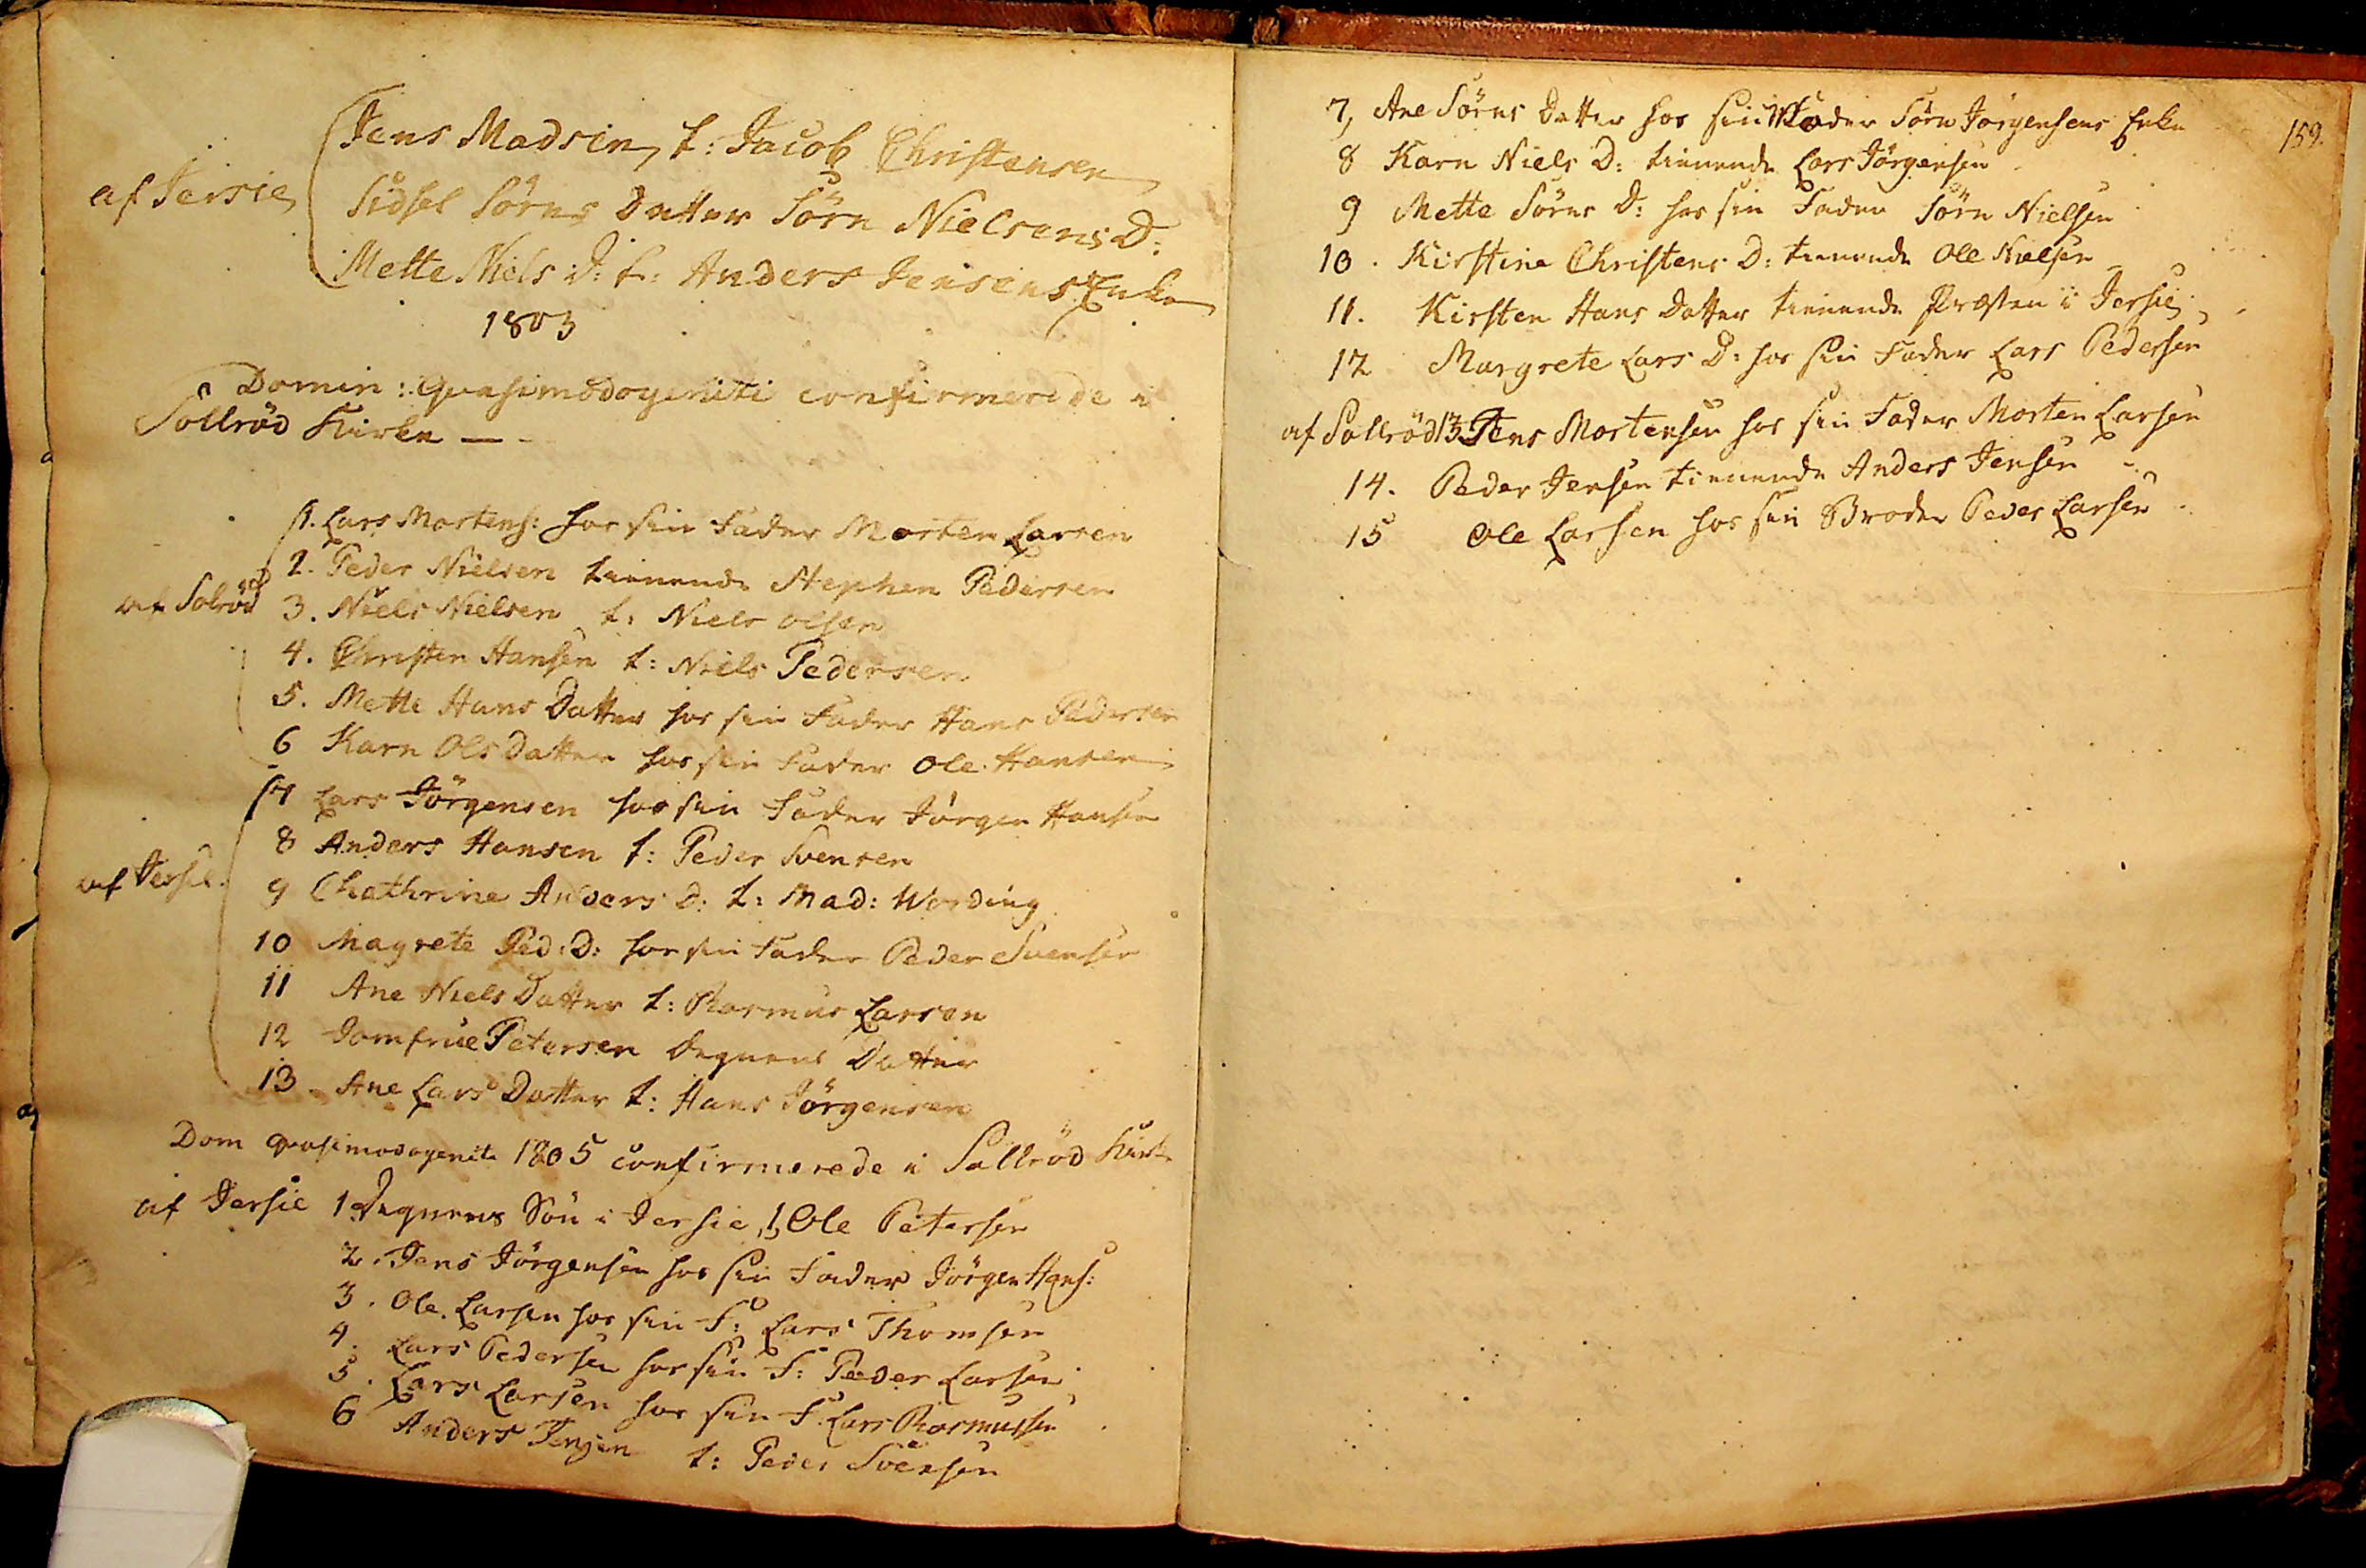Jens Madsen, t: Jacob Christensen
af Jersie Sidsel Sørns Datter Sørn Nielsens D:
Mette Niels D: t: Anders Jensens Enke
1803
Domin: Qvasimodogeniti Confirmerede i
Sollrød Kirke --
1. Lars Mortens: hos sin Fader Morten Larsen
2. Peder Nielsen tienende Stephen Pedersen
af Solrød 3. Niels Nielsen t: Niels Olsen
4. Christen Hansen t: Niels Pedersen
5. Mette Hans Datter hos sin Fader Hans Pedersen
6 Karn Ols Datter hos sin Fader Ole Hansen
7 Lars Jørgensen hos sin Fader Jørgen Hansen
8 Anders Hansen t: Peder Svensen
af Jersie 9. Chathrine Anders D: t: Mad: Wording
10. Magrete Ped: D: hos sin Fader Peder Svensen
11 Ane Niels Datter t: Rasmus Larsen
12 Jomfrue Petersen Degnens Datter
13. Ane Lars Datter t: Hans Jørgensen
Dom Qvasimodogeniti 1805 Confirmerede i Sollrød Kirke
af Jersie 1. Degnens Søn i Jersie, 1, Ole Petersen
2. Jens Jørgensen hos sin Fader Jørgen Hans:
3. Ole Larsen hos sin F: Lars Thomsen
4. Lars Pedersen hos sin F: Peder Larsen
5. Lars Larsen hos sin F: Lars Rasmussen
6. Anders Jensen t: Peder Svensen
159.
7, Ane Sørns Datter hos sin Moder Sørn Jørgensens Enke
8 Karn Niels D: tienende Lars Jørgensen
9 Mette Sørns D: hos sin Fader Sørn Nielsen
10. Kirsten Christens D: tienende Ole Nielsen
11. Kirsten Hans Datter tienende Præsten i Jersie
12. Margrete Lars D: hos sin Fader Lars Pedersen
af Solrød 13 Jens Mortensen hos sin Fader Morten Larsen
14. Peder Jensen tienende Anders Jensen
15 Ole Larsen hos sin Broder Peder Larsen
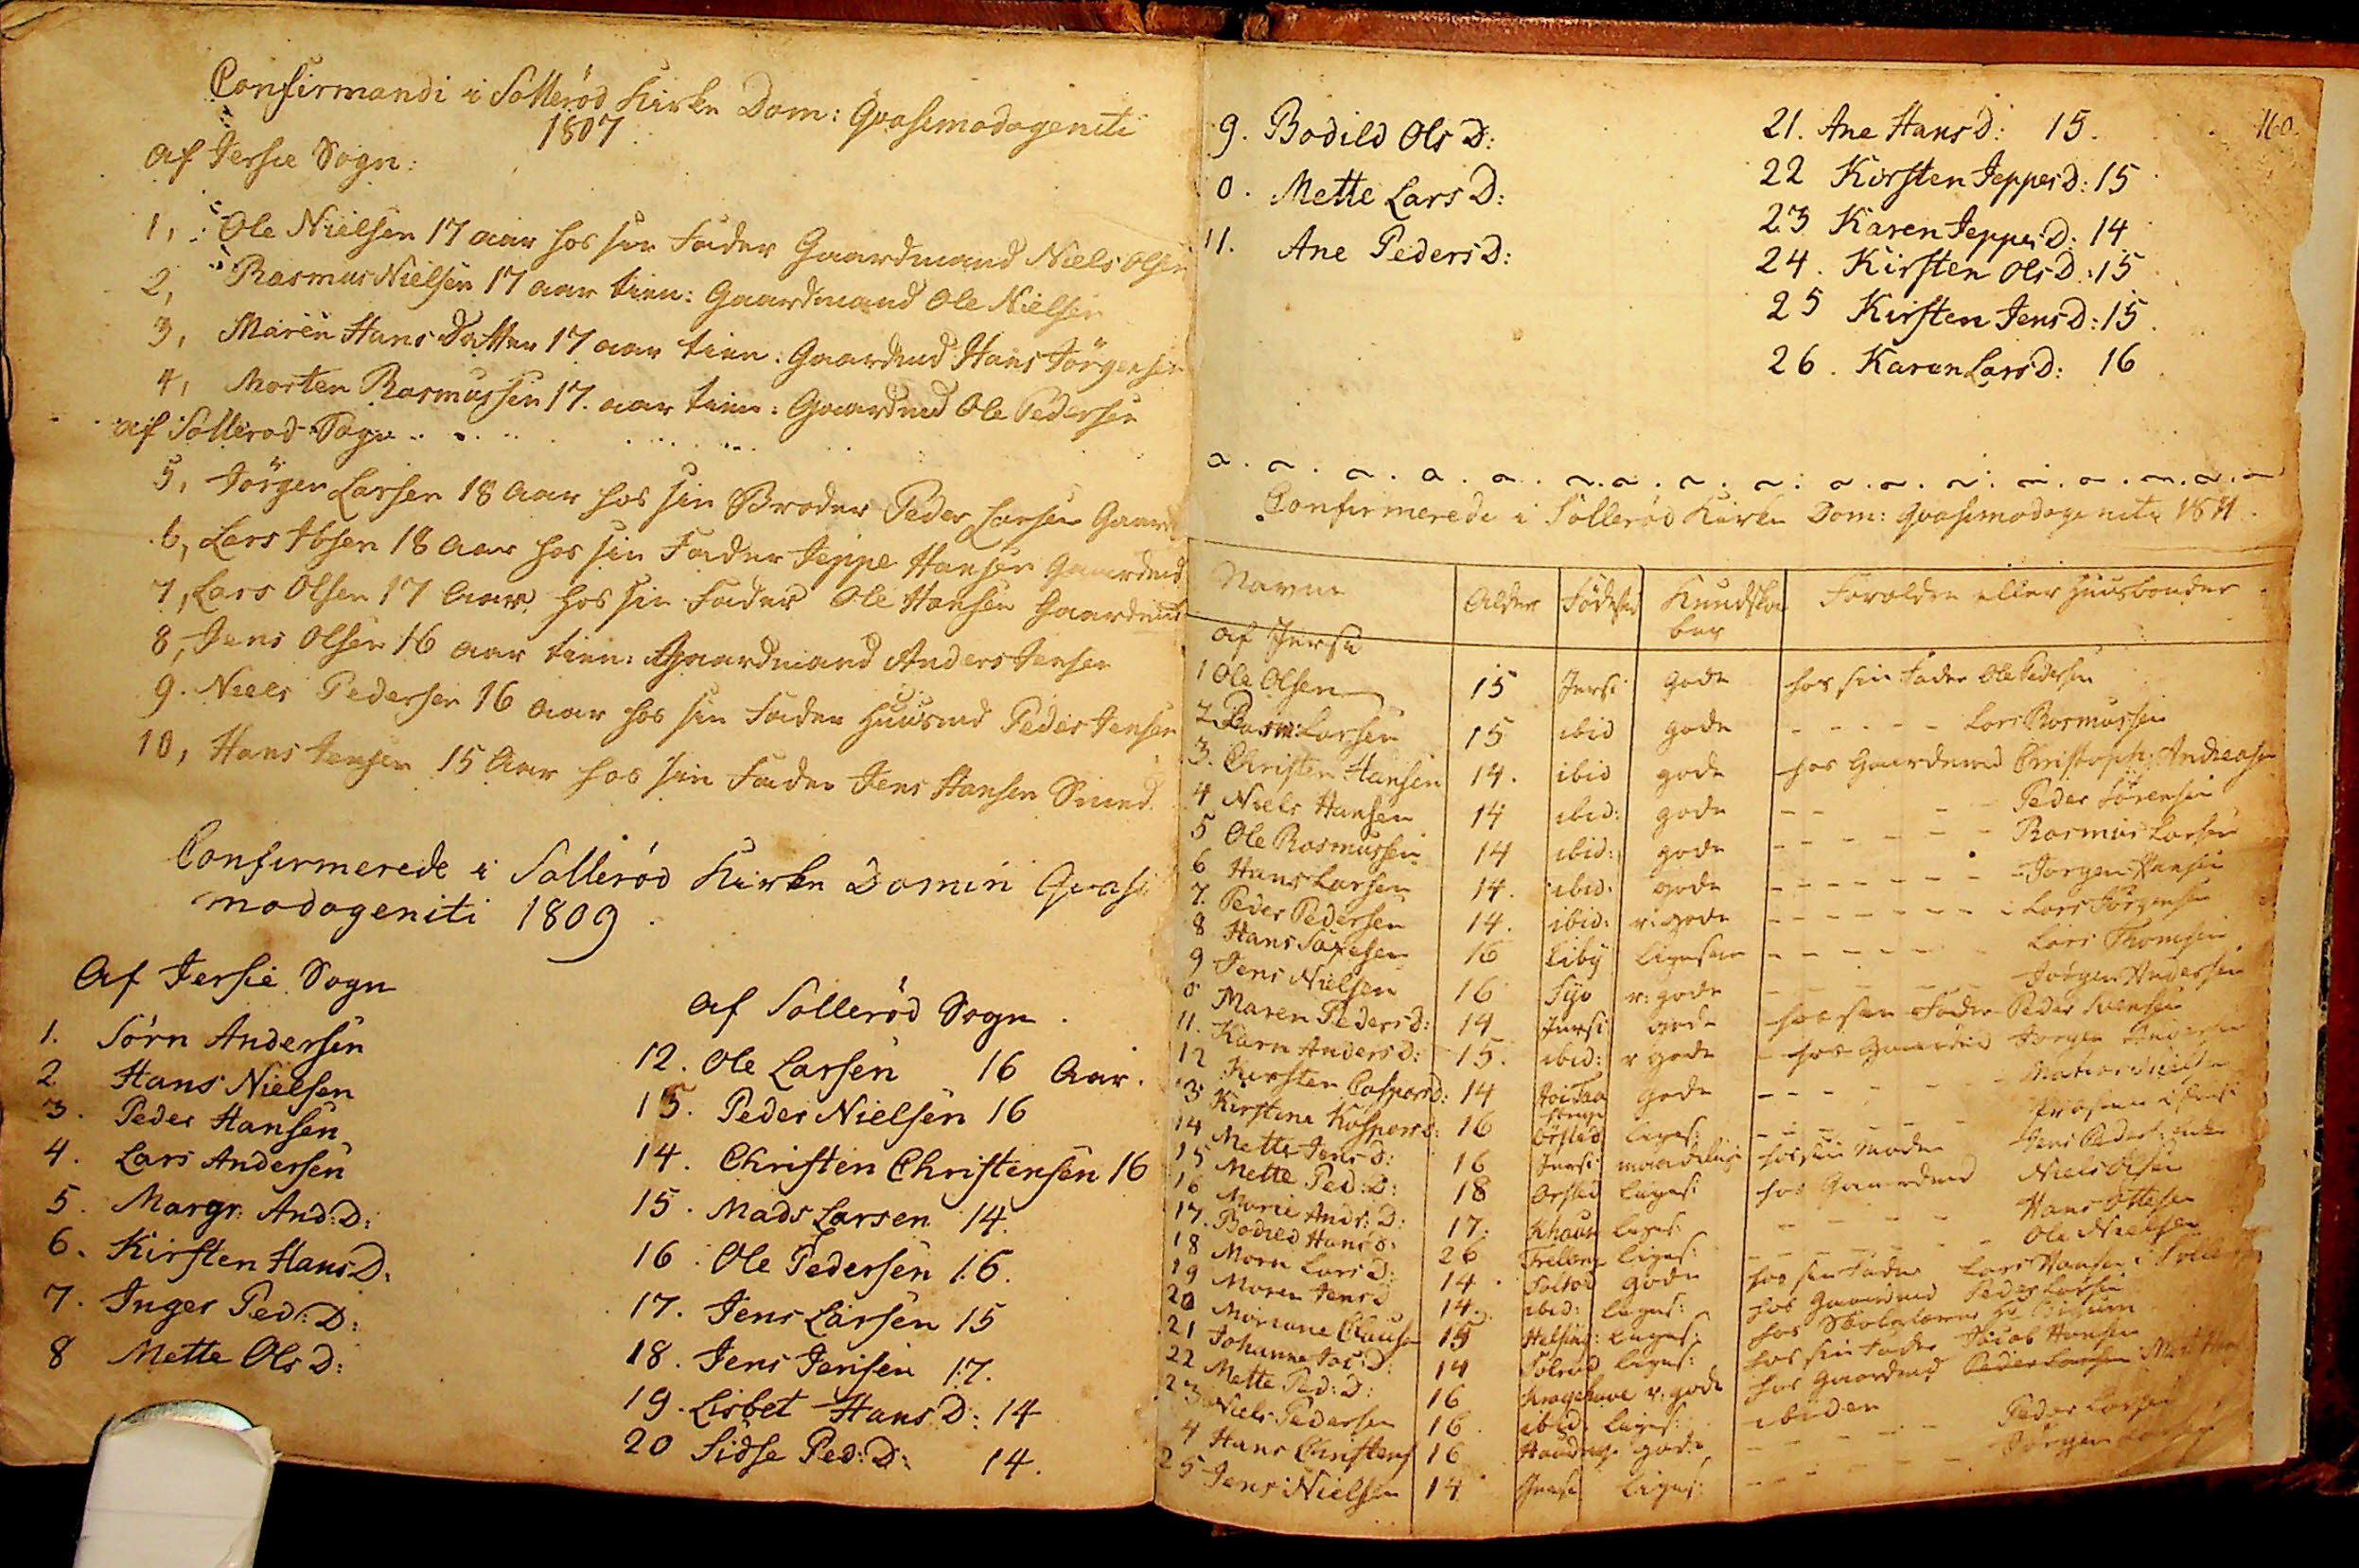Confirmerede i Sollerød Kirke Dom: Qvasimodogeniti
1807.
Af Jersie Sogn:
1, Ole Nielsen 17 Aar hos sin Fader Gaardmand Niels Olsen
2, Rasmus Nielsen 17 Aar tien: Gaardmand Ole Nielsen
3, Maren Hans Datter 17 Aar tien: Gaardmd Hans Jørgensen
4, Morten Rasmussen 17 Aar tien: Gaardmd Ole Pedersen
af Sollerød Sogn
5, Jørgen Larsen 18 Aar hos sin Broder Peder Larsen Gaard
6, Lars Ibsen 18 Aar hos sin Fader Jeppe Hansen Gaardmd
7, Lars Olsen 17 Aar hos sin Fader Ole Hansen Gaardmd
8, Jens Olsen 16 Aar tien: Gaardmand Anders Jensen
9, Niels Pedersen 16 Aar hos sin Fader Huusmd Peder Jensen
10, Hans Jensen 15 Aar hos sin Fader Jens Hansen Smed.
Confirmerede i Sollerød Kirke Domin Qvasi
modogeniti 1809.
Af Jersie Sogn
1. Sørn Andersen
2. Hans Nielsen
3. Peder Hansen
4. Lars Andersen
5. Margr: And:D:
6. Kirsten HansD.
7. Inger Ped:D:
8 Mette Ols D:
af Sollerød Sogn.
12. Ole Larsen 16 Aar
13. Peder Nielsen 16
14. Christen Christensen 16
15. Mads Larsen 14
16. Ole Pedersen 16
17. Jens Larsen 15
18. Jens Jensen 17
19. Lisbet Hans D: 14
20 Sidse Ped:D: 14
9. Bodild Ols D:
0. Mette Lars D:
11. Ane PedersD:
160.
21. Ane Hans D: 15
22 Kirsten Jeppes D: 15
23 Karen Jeppes D: 14
24. Kirsten Ols D: 15
25 Kirsten Jens D: 15
26. Karen Lars D: 16
Confirmerede i Sollerød Kirke Dom: Qvasimodogeniti 1811
Navne
Alder
Fødested
Kundska
ber
Forældre eller Huusbonden
af Jersi
1 Ole Olsen
2 Rasm: Larsen
3. Christen Hansen
4 Niels Hansen
5 Ole Rasmussen
6 Hans Larsen
7. Peder Pedersen
8 Hans Sørensen
9 Jens Nielsen
0 Maren PedersD:
11. Karens AndersD:
12 Kirsten CasporD:
13 Kirsen KasporD:
14 Mette JensD:
15 Mette Ped:D:
16 Marie Ands:D:
17. Bodild HansD:
18 Marn LarsD:
19 Marn JensD
20 Mariane Clausen
21 Johanne Jac:D:
22 Mette Ped:D:
23 Niels Pedersen
4 Hans Christens
25 Jens Nielsen
15
15
14
14
14
14.
14.
16
16
14
15
14
16
16
18
17.
26
14
14
15
14
16
16
16
14
Jersi
ibid
ibid
ibid:
ibid:
ibid.
ibid:
Eiby
Syv
Jersi
ibid:
Hoi Taa
strup
Ørsted
Jersi
Ørsted
Khaun
Trellerup
Solrød
ibid:
Helsing:
Solrød
Kragehave
ibid
Haudrup
Jersi
gode
gode
gode
gode
gode
gode
r:gode
Ligesaa
r:gode
gode
r gode
gode
liges:
maadelig
Liges:
Liges:
Liges:
gode
Liges:
Liges:
Liges:
r:gode
Ligel:
gode
Ligel:
hos sin fader Ole Pedersen
Lars Rasmussen
hos Gaardmand Christoph: Andreasen
Peder Sørensen
Rasmus Larsen
Jørgen Hansen
Lars Jørgensen
Lars Thomsen
Jørgen Andersen
hos sin Fader Peder Svensen
hos Gaardmd Jørgen Andersen
Morten Nielsen
Præsten i Jersi
hos sin Moder Jens Peders Enke
hos Gaardmd Niels Olsen
Hans Ottesen
Ole Nielsen
hos sin Fader Lars Hansen i Solrød
hos Gaardmand Peder Larsen
hos Skolelære Hr Bigum
hos sin Fader Jacob Hansen
hos Gaardmd Peder Larsen Morten:Hans:
ibidem
Peder Larsen
Jørgen Larsen